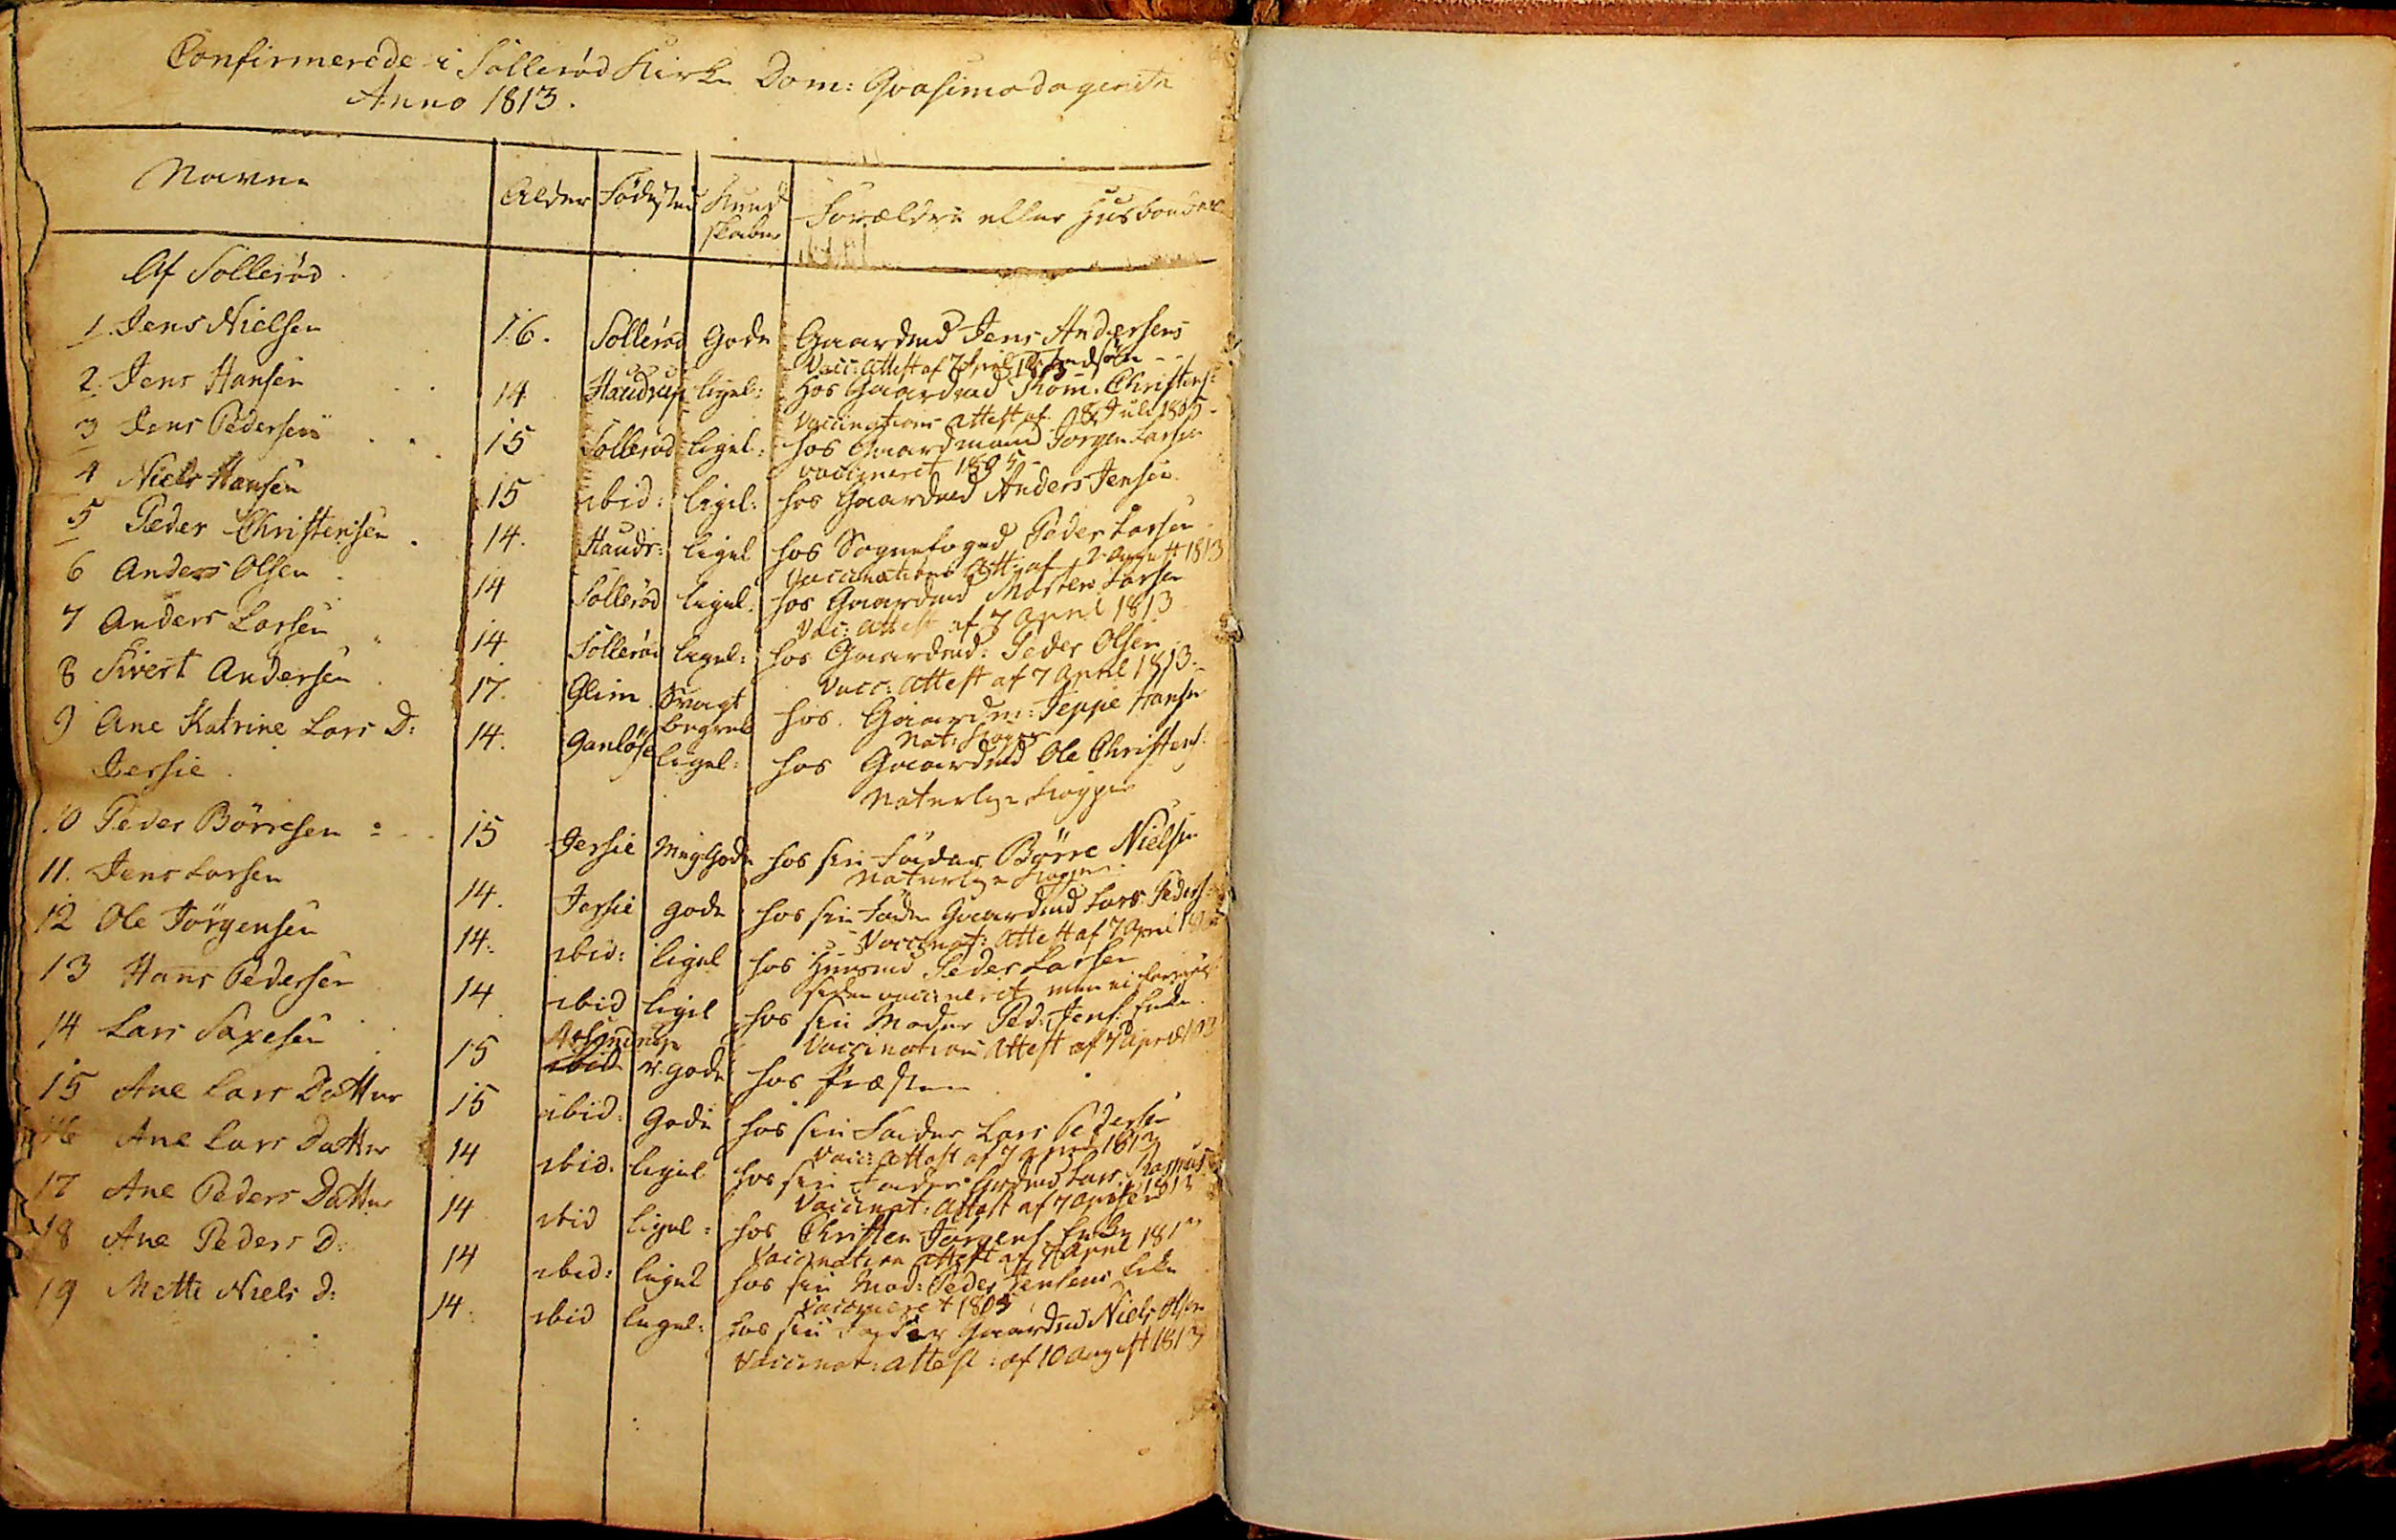Confirmerede i Sollerød Kirke Dom: Qvasimodogeniti
Anno 1813.
Navne
Alder
Fødested
Kund
skaber
Forældre eller Husbonden
Af Sollerød
1. Jens Nielsen
2. Jens Hansen
3 Jens Pedersen
4 Niels Hansen
5 Peder Christensen
6 Anders Olsen
7 Anders Larsen
8 Sivert Andersen
9 Ane Katrine Lars D:
Jersie
10 Peder Børresen
11 Jens Larsen
12 Ole Jørgensen
13 Hans Pedersen
14 Lars Saxesen
15 Ane Lars Datter
16 Ane Lars Datter
17 Ane Peders Datter
18 Ane Peders D:
19 Mette Niels D:
16.
14
15
15
14
14
14
17
14.
15
14.
14.
14
15
15
14
14
14
14
Sollerød
Haudrup
Sollerød
ibid:
Haudr:
Sollerød
Sollerød
Glim
Ganløse
Jersie
Jersie
ibid:
ibid:
Assendrup
ibid
ibid:
ibid.
ibid
ibid:
ibid
gode
ligel:
ligel:
ligel:
ligel:
ligel:
ligel:
Svagt
begavet
ligel:
meg:gode
gode
ligel
ligel
r.gode
gode
ligel:
ligel
ligel:
ligel:
Gaardmd Jens Andersens
Vacc:Attest af 7 April 1813 ##
Hos Gaardmd Rasm:Christens:
Vaccination Attest af 8 Juli 1805
hos Gaardmand Jørgen Larsen
vaccineret 1805
hos Gaardmd Anders Jensen
hos Sognefoged Peder Larsen
Vaccinations Att: af 2 August 1813
hos Gaardmd Morten Larsen
Vac: Attest af 7 April 1813
hos Gaardmd: Peder Olsen
Vacc: Attest af 7 April 1813.
hos Gaardm: Jeppe Hansen
Nat: Kopper
hos Gaardmd Ole Christens:
Naturlige Kopper
hos sin Fader Børre Nielsen
Naturlige Kopper
hos sin Fader Gaardmd Lars Peders:
Vaccinat: Attest af 7 April 1813
hos Huusmd Peder Larsen
siden vaccineret men ei forevist
hos sin Moder Ped: Jens: Enke
Vaccinations Attest af 7 April 1813
hos Præsten
hos sin Fader Lars Pedersen
Vacc: Attest af 7 April 1813
hos sin Fader Grdmd Lars Rasmus:
Vaccinat. Attest af 7 April 1813
hos Christen Jørgens: Enke
Vaccination Attest af 7 April 1813
hos sin Mod: Peder Jensens Enke
Vaccineret 1805
hos sin Fader Gardmd Niels Olsen
Vaccinat: Attest af 10 August 1813